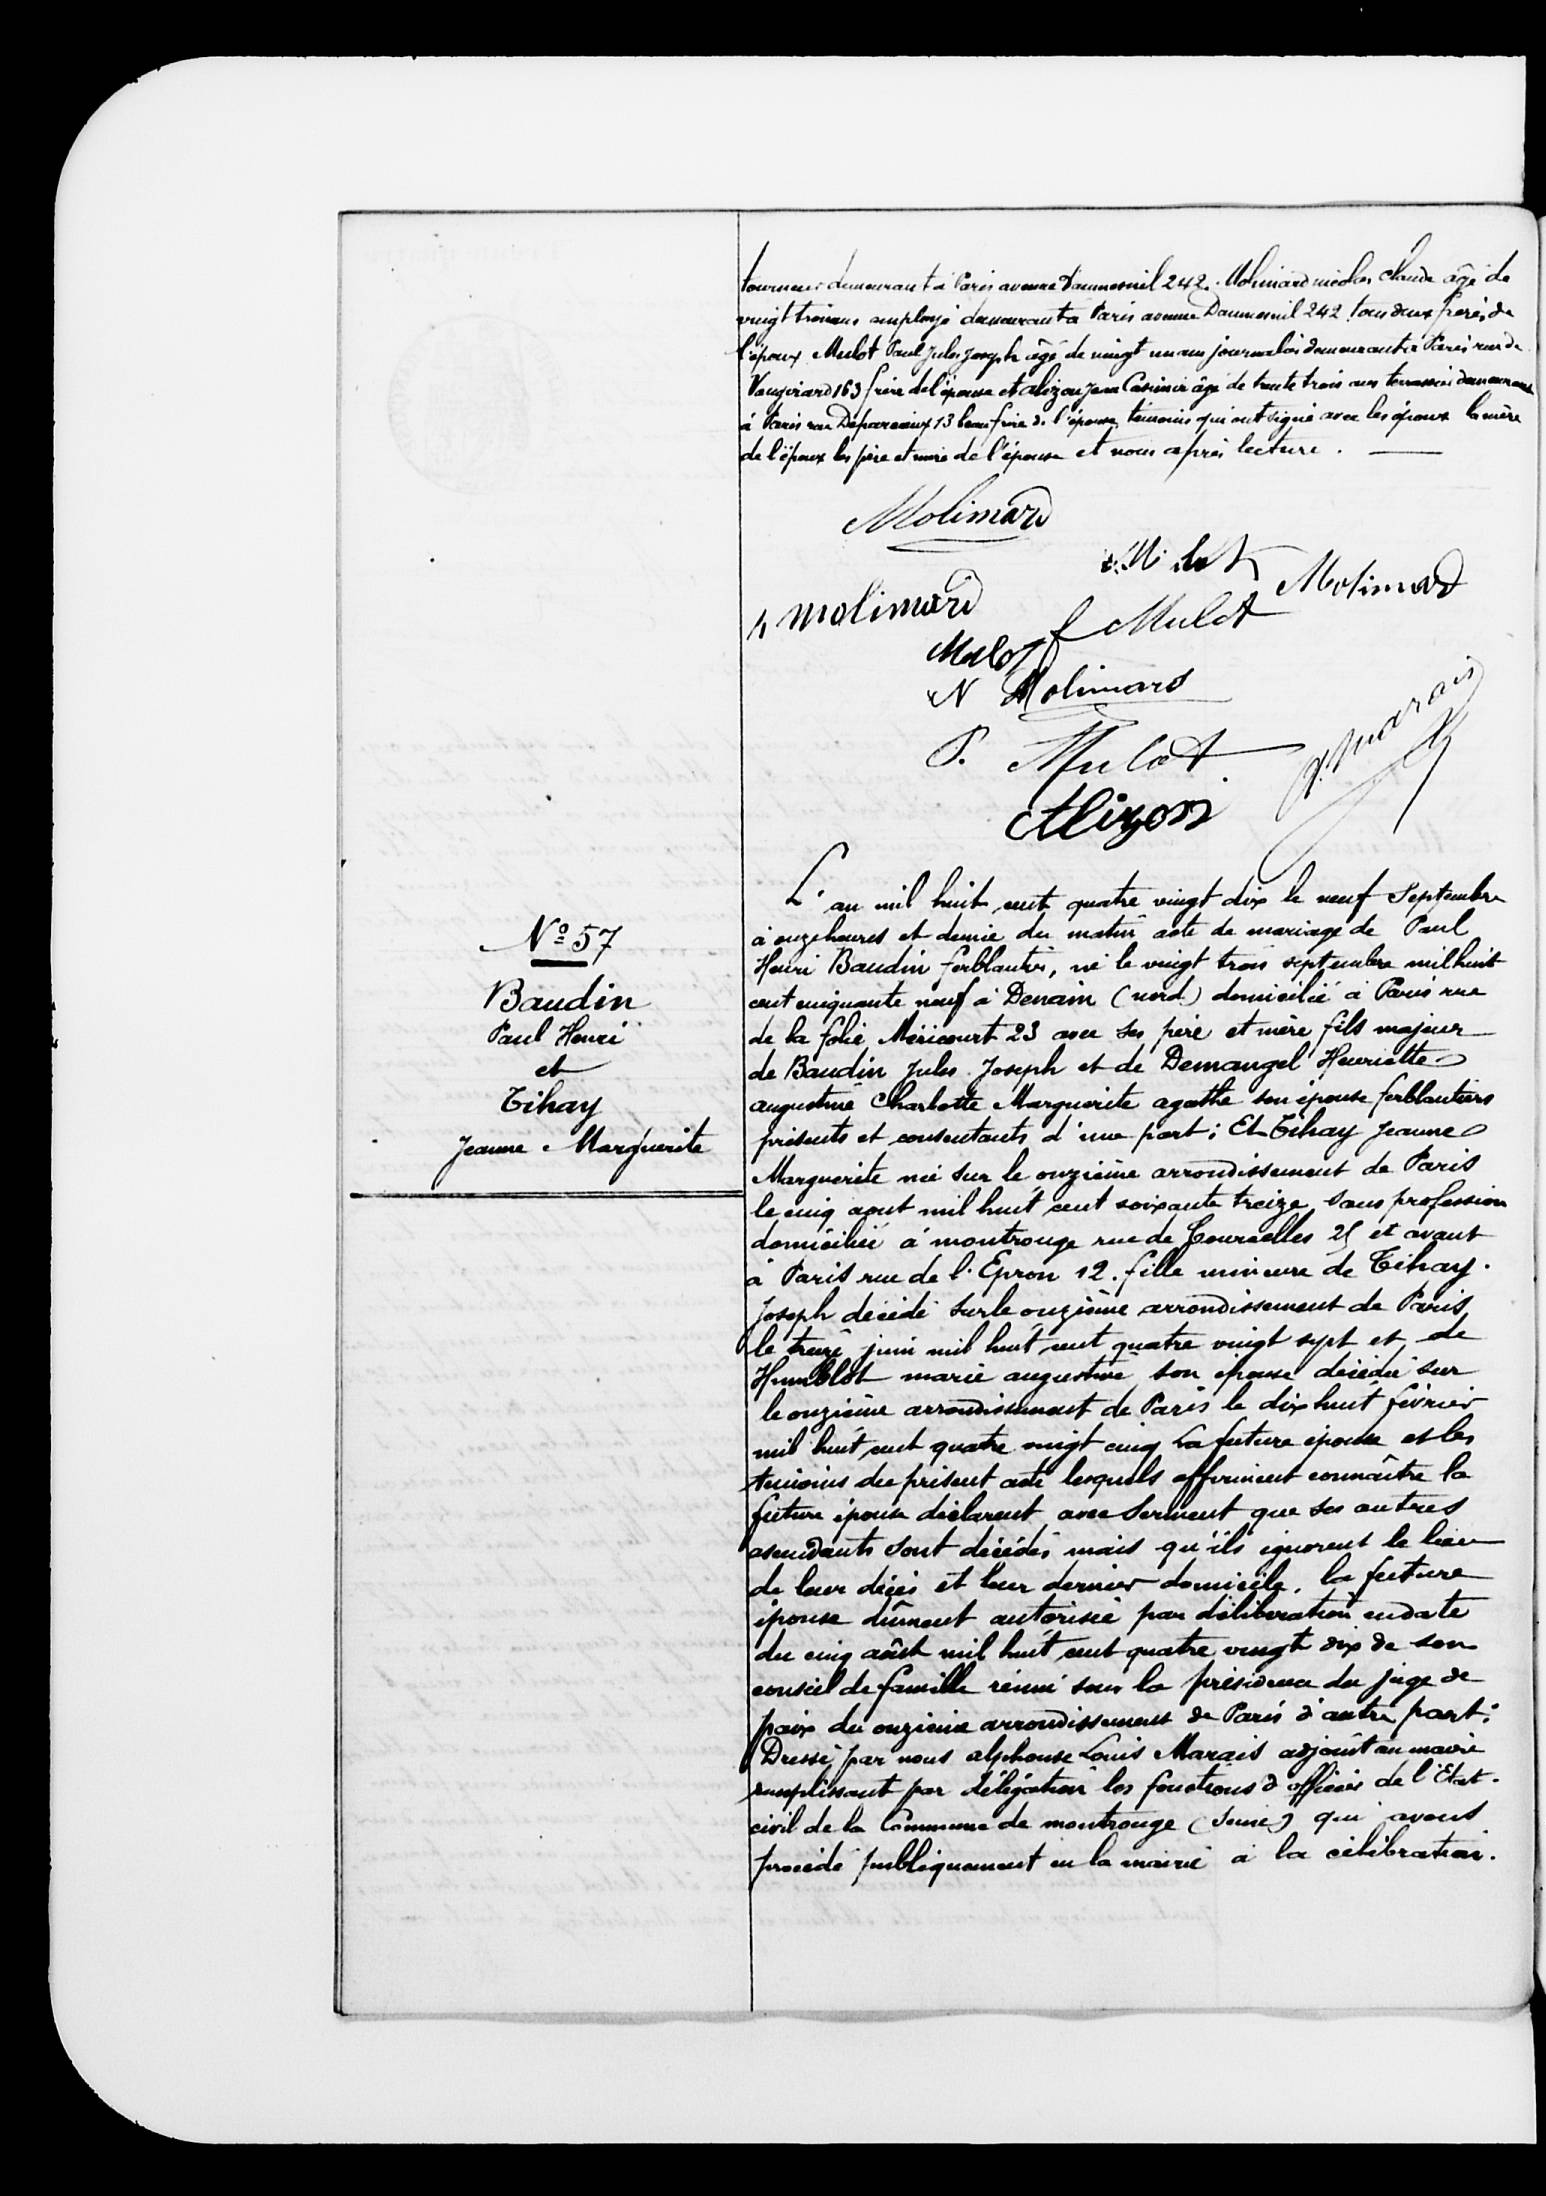tourneur demeurant à Paris avenue Daumesnil 242 Moliniard méolo, claude âgé de
vingt trois ans, employé demeurant à Paris avenue Daumesnil 242 tous deux frère de
l'époux Mulot Paul Julien Joseph âgé de vingt un ans journalier demeurant à Paris rue de
Vaugirar 169 frère del'épouse et alizou Jean Carinier âgé de toute trois ans français demeurant
à Paris en Depereaux 13 beau frère de l'épouse, témoins qui ont signé avec les époux la mère
de l'épouse les père et mère de l'épouse et nous après lecture. -
N°57
Baudin
Paul Henri
et
Tihay
Jeanne Marguerite
L'an mil huit cent quatre vingt dix le neuf Septembre
à onze heures et demie du matin acte de mariage de Paul
Henri Baudin Ferblautin, né le vingt trois septembre mil huit
cent cinquante neuf à Denaim (nord) domicilié à Paris rue
de la folie Méricourt 23 avec ses père et mère fils majeur
de Baudin Jules Joseph et de Demaugel Henriette
augustine Charlotte Marguerite agathe sur épouse fablautine
présents et consentants, d'une part; Et Tihay Jeanne
Marguerite née sur le onzième arrondissement de Paris
le cinq aout mil huit cent soixante treize sans profession
domiciliée à montrouge rue de Courcelles 25 et avant
à Paris rue de l'Epron 12. fille mineur de Tihay.
Joseph décédé sur le onzième arrondissement de Paris
le treize juin mil huit cent quatre vingt sept et de
Humblot marie augustine son épouse décédée sur
le onzième arrondissement de Paris le dix huit février
mil huit cent quatre vingt cinq la future épouse et les
témoins du présent acte lesquels affirmaent connaître la
future épouse déclarent avec serment que ses autres
ascendants sont décédés, mais qu'ils ignorent le lieu
de leur décès et leur dernier domicile, la future
épouse dûment autorisée par délibération en date
du cinq août mil huit cent quatre vingt deux de son
conseil de famille résumé sous la présence du juge de
passé du onzième arrondissement de Paris d'autre part:
Dressé par nous alphonse Louis Marais adjoint au maire
remplissant par délégation les fonctions d'officier de l'Etat-
civil de la Commune de montrouge (Seine) qui avons
procédé publiquement en la mairie à la célébration.
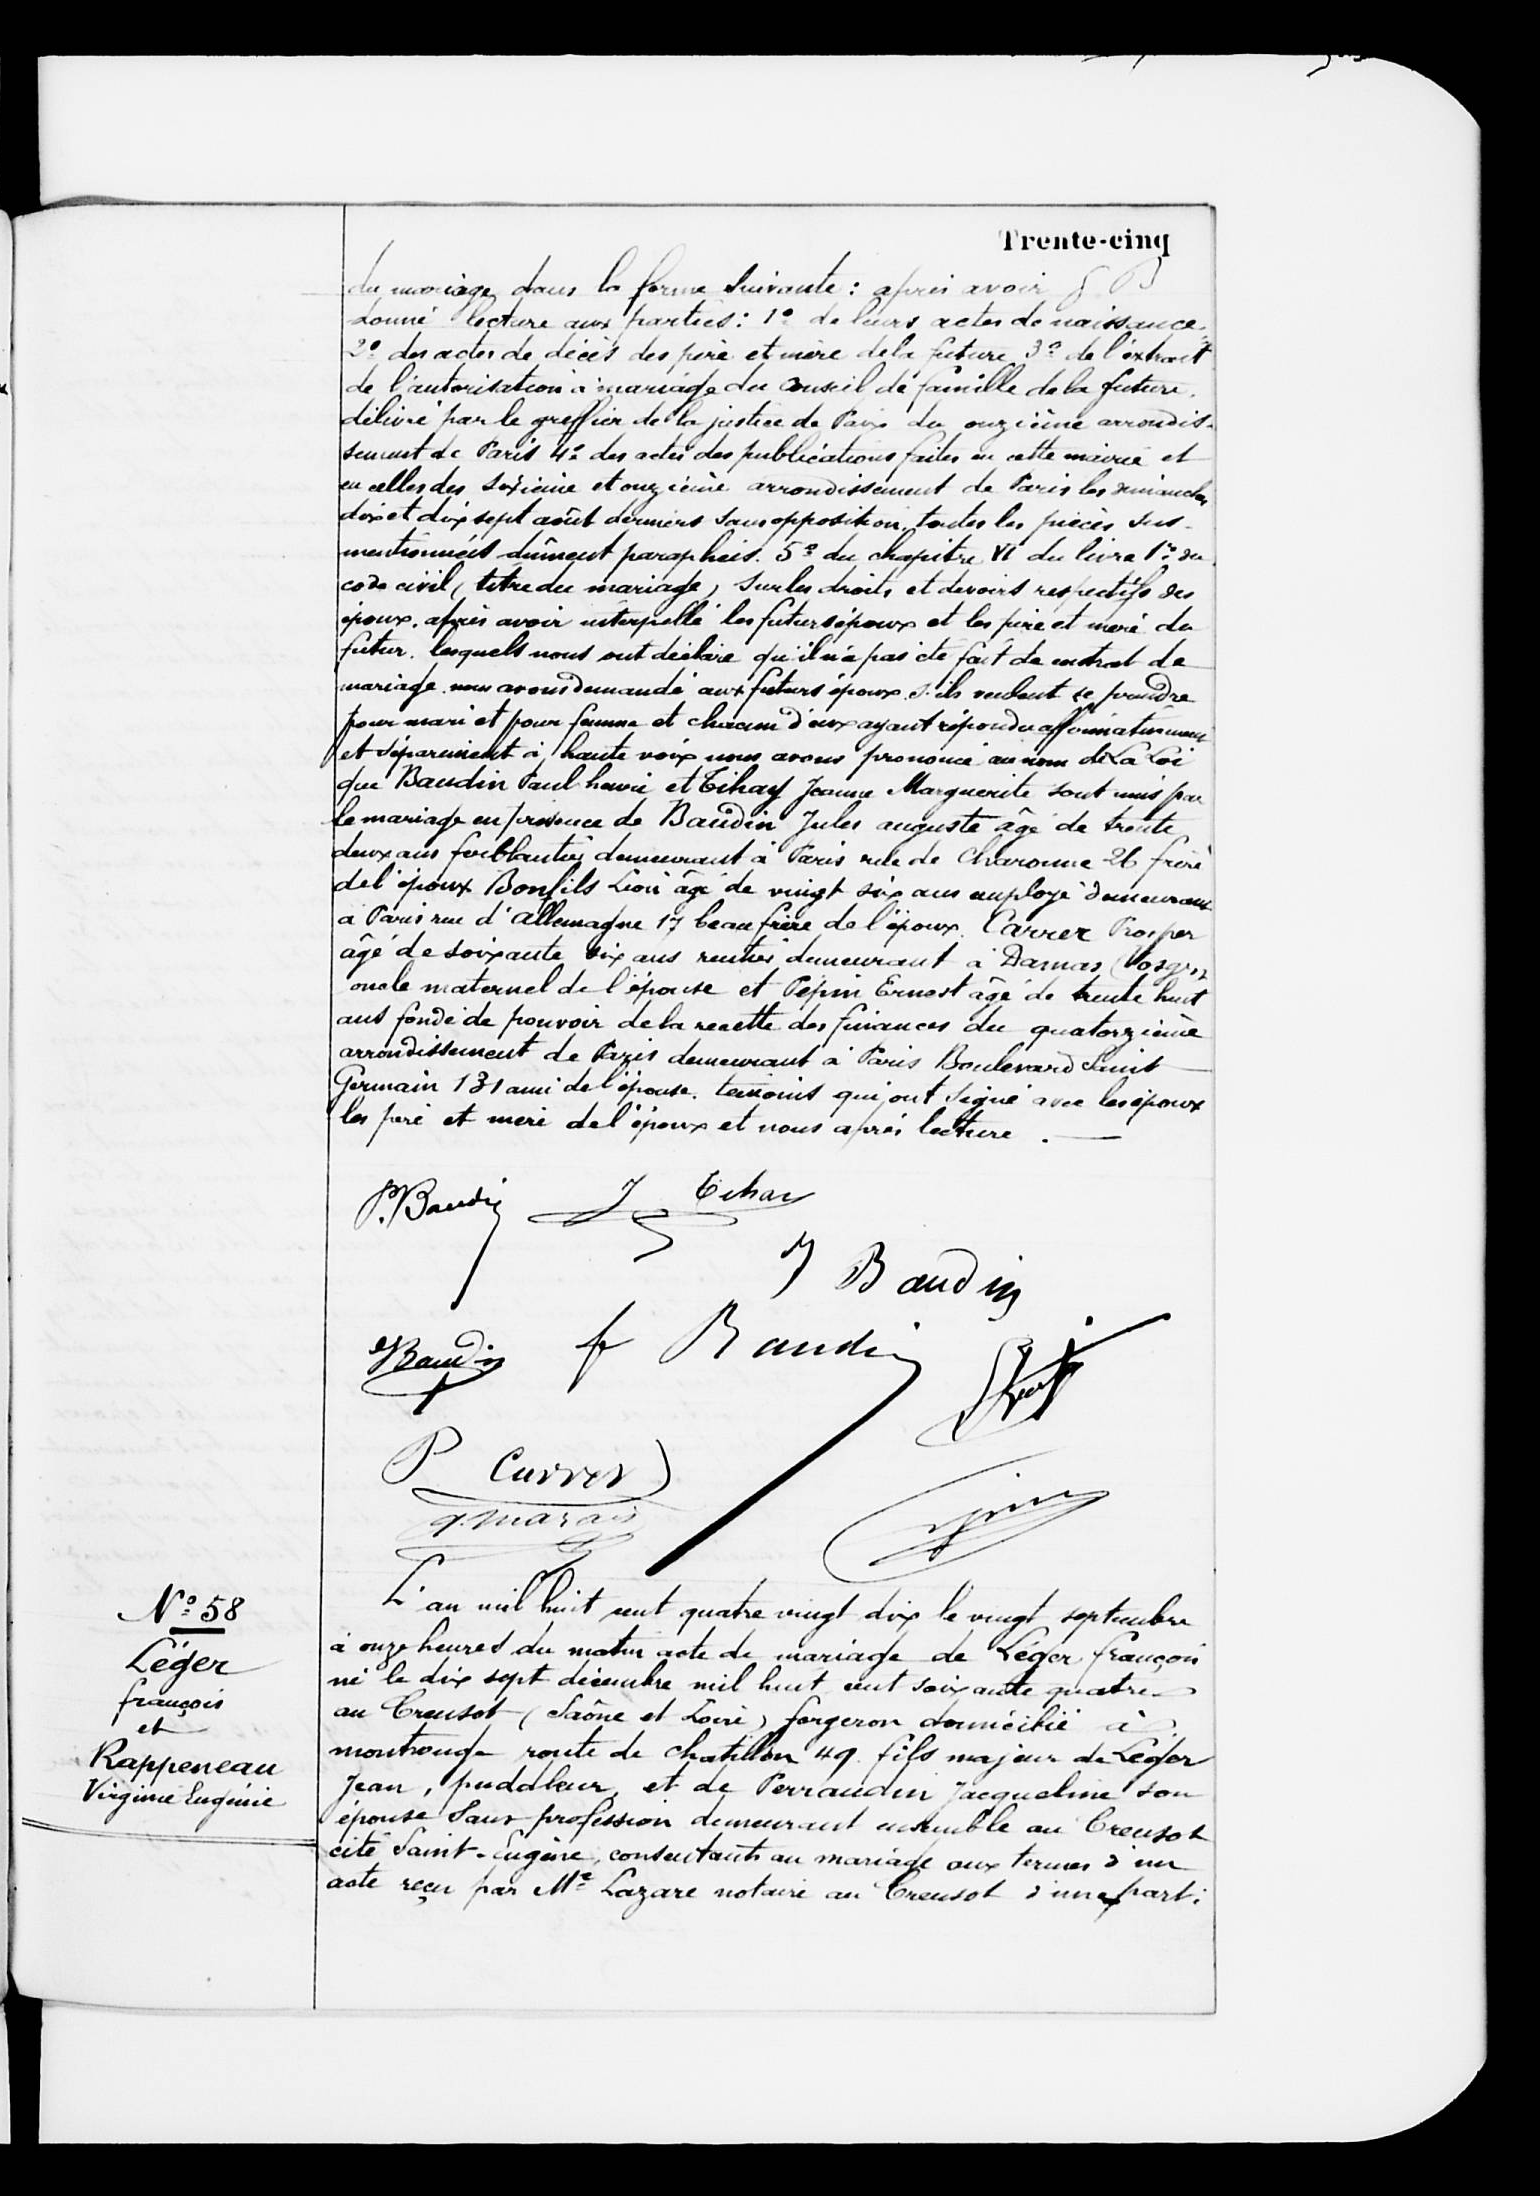du mariage dans la forme suivant: après avoir
donné lecture aux parties: 1°: de leurs actes de naissance
2°: des actes de décès du père et mère de la future 3° de l'extrait
de l'autrisation à mariage du conseil de famille de la future
délivré par le freffier de la justice de Paris la onzième arrondis-
sement de Paris 4°: Les actes de publication faites en cette mairie et
en celles des septième et onzième arrondissement de Paris les mercredi
trois et dix sept août derniers sous oppositions toutes les pièces sus-
mntionnées dûment paraphées. 5°: du chapitre VI du livre 1r dr
code civil (titre du mariage) sur les droits et devoirs respectifs des
époux après avoir interpellé les futurs époux et les père et mère la
futur lesquels nous on tdéclaré qu'il n'a pas été fait de contrat de
mariage nous avons demandé aux futurs époux s'ils veulent se prendre
pour mari et pour femme et chacun d'eux ayant répondu affirmativement
et séparément à haute voix nous avons prononcé au nom de La Loi
que Baudin Paul Bauvie et Tihay Jeanne Marguerite sont unis par
le mariage en présence de Baudin Jules auguste âgé de trente
deux ans fablautier demeurant à Paris rue de charonne 26 frère
del'époux Bonfils Léon âgé de vingt six ans employé de commerce
à Paris rue d'allemagne 17 beau frère de l'époux Carrer Prosper
âgé de soixante six ans rentier demeurant à Darnas (Vosges)
oncle maternel de l'épouse et Pepin Ernest âgé de trente huit
ans fondé de pouvoir de la recette des finances du quatorzième
arrondissement de Paris demeurant à Paris Boulevard Saint
Germain 131 ami de l'épouse toujours qui ont signé avec les époux
les père et mère de l'époux et nous après lecture.-
N°58
Léger
françois
et
Rappeneau
Viergine Eugénie
L'an mil huit cent quatre vingt dix le vingt septembre
à onze heure du matin acte de mariage de Léger Frauçon
né le dix sept décembre mil huit cent soixante quatre
au Creusot (Saône et Loire) forgeron domicilié à
moutrouge route de Chatillon 49 fils majeur de Lego
Jean puddleur et de Perraudin Jacqueline son
épouse sans profession demeurant ensemble au Creusot
cité Saint-Eugène, contractants au mariage aux termes d'un
acte reçu par Me Lazare notaire au Creusot d'un part.